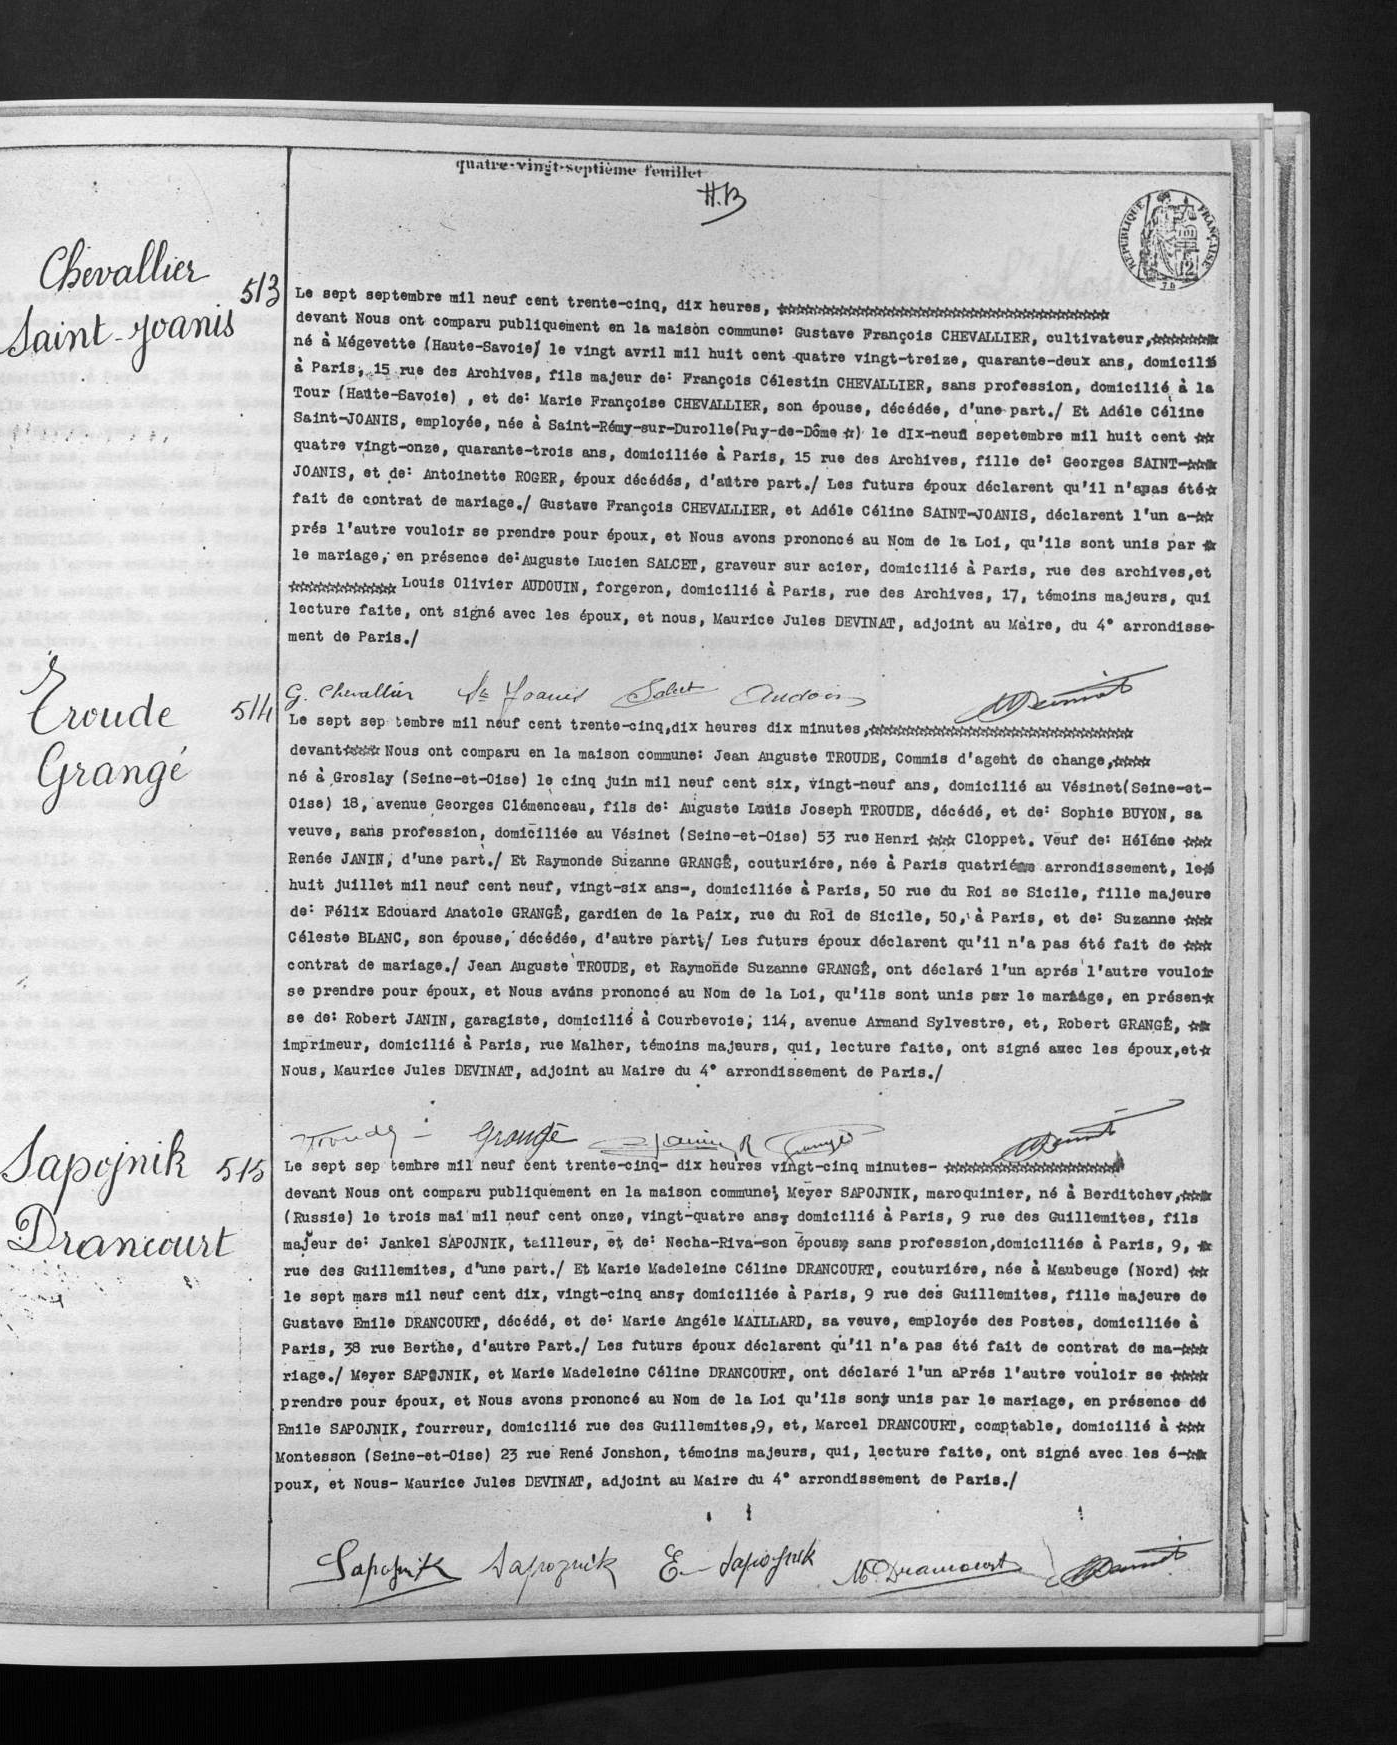Le sept septembre mil neuf cent trente-cinq, dix heures, ****
devant Nous ont comparu publiquement en la maison commune: Gustave François CHEVALLIER, cultivateur, ****
né à Mégevette (Haute-Savoie) le vingt avril mil huit cent quatre vingt-treize, quarante-deux ans, domicilié
à Paris, 15 rue des Archives, fils majeur de: François Célestin CHEVALLIER, sans profession, domicilié à la
Tour (Haute-Savoie), et de: Marie Françoise CHEVALLIER, son épouse, décédée, d'une part ./ Et Adèle Céline
Saint-JOANIS, employée, née à Saint-Rémy-sur-Durolle (Puy-de-Dôme *) le dIx-neuf sepetembre mil huit cent **
quatre vingt-onze, quarante-trois ans, domiciliée à Paris, 15 rue des Archives, fille de: Georges SAINT -***
JOANIS, et de: Antoinette ROGER, époux décédés, d'autre part ./ Les futurs époux déclarent qu'il n'a pas été*
fait de contrat de mariage ./ Gustave François CHEVALLIER, et Adéle Céline SAINT-JOANIS, déclarent l'un a -**
prés l'autre vouloir se prendre pour époux, et Nous avons prononcé au Nom de la Loi, qu'ils sont unis par *
le mariage; en présence de: Auguste Lucien SALCET, graveur sur acier, domicilié à Paris, rue des archives,et
**** Louis Olivier AUDOUIN, forgeron, domicilié à Paris, rue des Archives, 17, témoins majeurs, qui
lecture faite, ont signé avec les époux, et nous, Maurice Jules DEVINAT, adjoint au Maire, du 4° arrondisse-
ment de Paris ./
Le sept sep tembre mil neuf cent trente-cinq, dix heures dix minutes ,****
devant *** Nous ont comparu en la maison commune: Jean Auguste TROUDE, Commis d'agent de change, ****
né à Groslay (Seine-et-Oise) le cinq juin mil neuf cent six, vingt-neuf ans, domicilié au Vésinet (Seine-et-
Oise) 18, avenue Georges Clémenceau, fils de: Auguste Louis Joseph TROUDE, décédé, et de: Sophie BUYON, sa
veuve, sans profession, domiciliée au Vésinet (Seine-et-Oise) 53 rue Henri *** Cloppet. Veuf de: Hélène ***
Renée JANIN, d'une part ./ Et Raymonde Suzanne GRANGE, couturière, née à Paris quatriéme arrondissement, le*
huit juillet mil neuf cent neuf, vingt-six ans -, domiciliée à Paris, 50 rue du Roi se Sicile, fille majeure
de: Félix Edouard Anatole GRANGE, gardien de la Paix, rue du Roi de Sicile, 50, à Paris, et de: Suzanne ***
Célest BLANC, son épouse, décédée, d'autre part ;/ Les futurs époux déclarent qu'il n'a pas été fait de ***
contrat de mariage ./ Jean Auguste TROUDE, et Raymonde Suzanne GRANGE, ont déclaré l'un aprés l'autre vouloir
se prendre pour époux, et Nous avons prononcé au Nom de la Loi, qu'ils sont unis par le mariage, en présen*
se de: Robert JANIN, garagiste, domicilié à Courbevoie, 114, avenue Armand Sylvestre, et, Robert GRANGE, **
imprimeur, domicilié à Paris, rue Malher, témoins majeurs, qui, lecture faite, ont signé avec les époux, et*
Nous, Maurice Jules DEVINAT, adjoint au Maire du 4° arrondissement de Paris ./
Le sept sep tembre mil neuf cent trente-cinq-dix heures vingt-cinq minutes -****
devant Nous ont comparu publiquement en la maison commune: Meyer SAPOJNIK, maroquinier, né à Berditchev, ***
(Russie) le trois mai mil neuf cent onze, vingt-quatre ans-domicilié à Paris, 9 rue des Guillemites, fils
majeur de: Jankel SAPOJNIK, taillleur, et de: Necha-Riva-son époux sans profession, domiciliés à Paris, 9, *
rue des Guillemites, d'une part ./ Et Marie Madeleine Céline DRANCOURT, couturiére, née à Maubeuge (Nord) **
le sept mars mil neuf cent dix, vingt-cinq ans, domiciliée à Paris, 9 rue des Guillemites, fille majeure de
Gustave Emile DRANCOURT, décédé, et de: Marie Angéle MAILLARD, sa veuve, employée des Postes, domiciliée à
Paris, 38 rue Berthe, d'autre Part ./ Les futurs époux déclarent qu'il n'a pas été fait de contrat de ma -***
riage ./ Meyer SAPOJNIK, et Marie Madelein Céline DRANCOURT, ont déclaré l'un aPrés l'autre vouloir se ****
prendre pour époux, et Nous avons prononcé au Nom de la Loi qu'ils sont unis par le mariage, en présence de
Emile SAPOJNIK, fourreur, domicilié rue des Guillemites, 9, et, Marcel DRANCOURT, comptable, domicilié à ***
Montesson (Seine-et-Oise) 23 rue René Jonshon, témoins majeurs, qui, lecture faite, ont signé avec les é -**
poux, et Nous-Maurice Jules DEVINAT, adjoint au Maire du 4° arrondissement de Paris ./